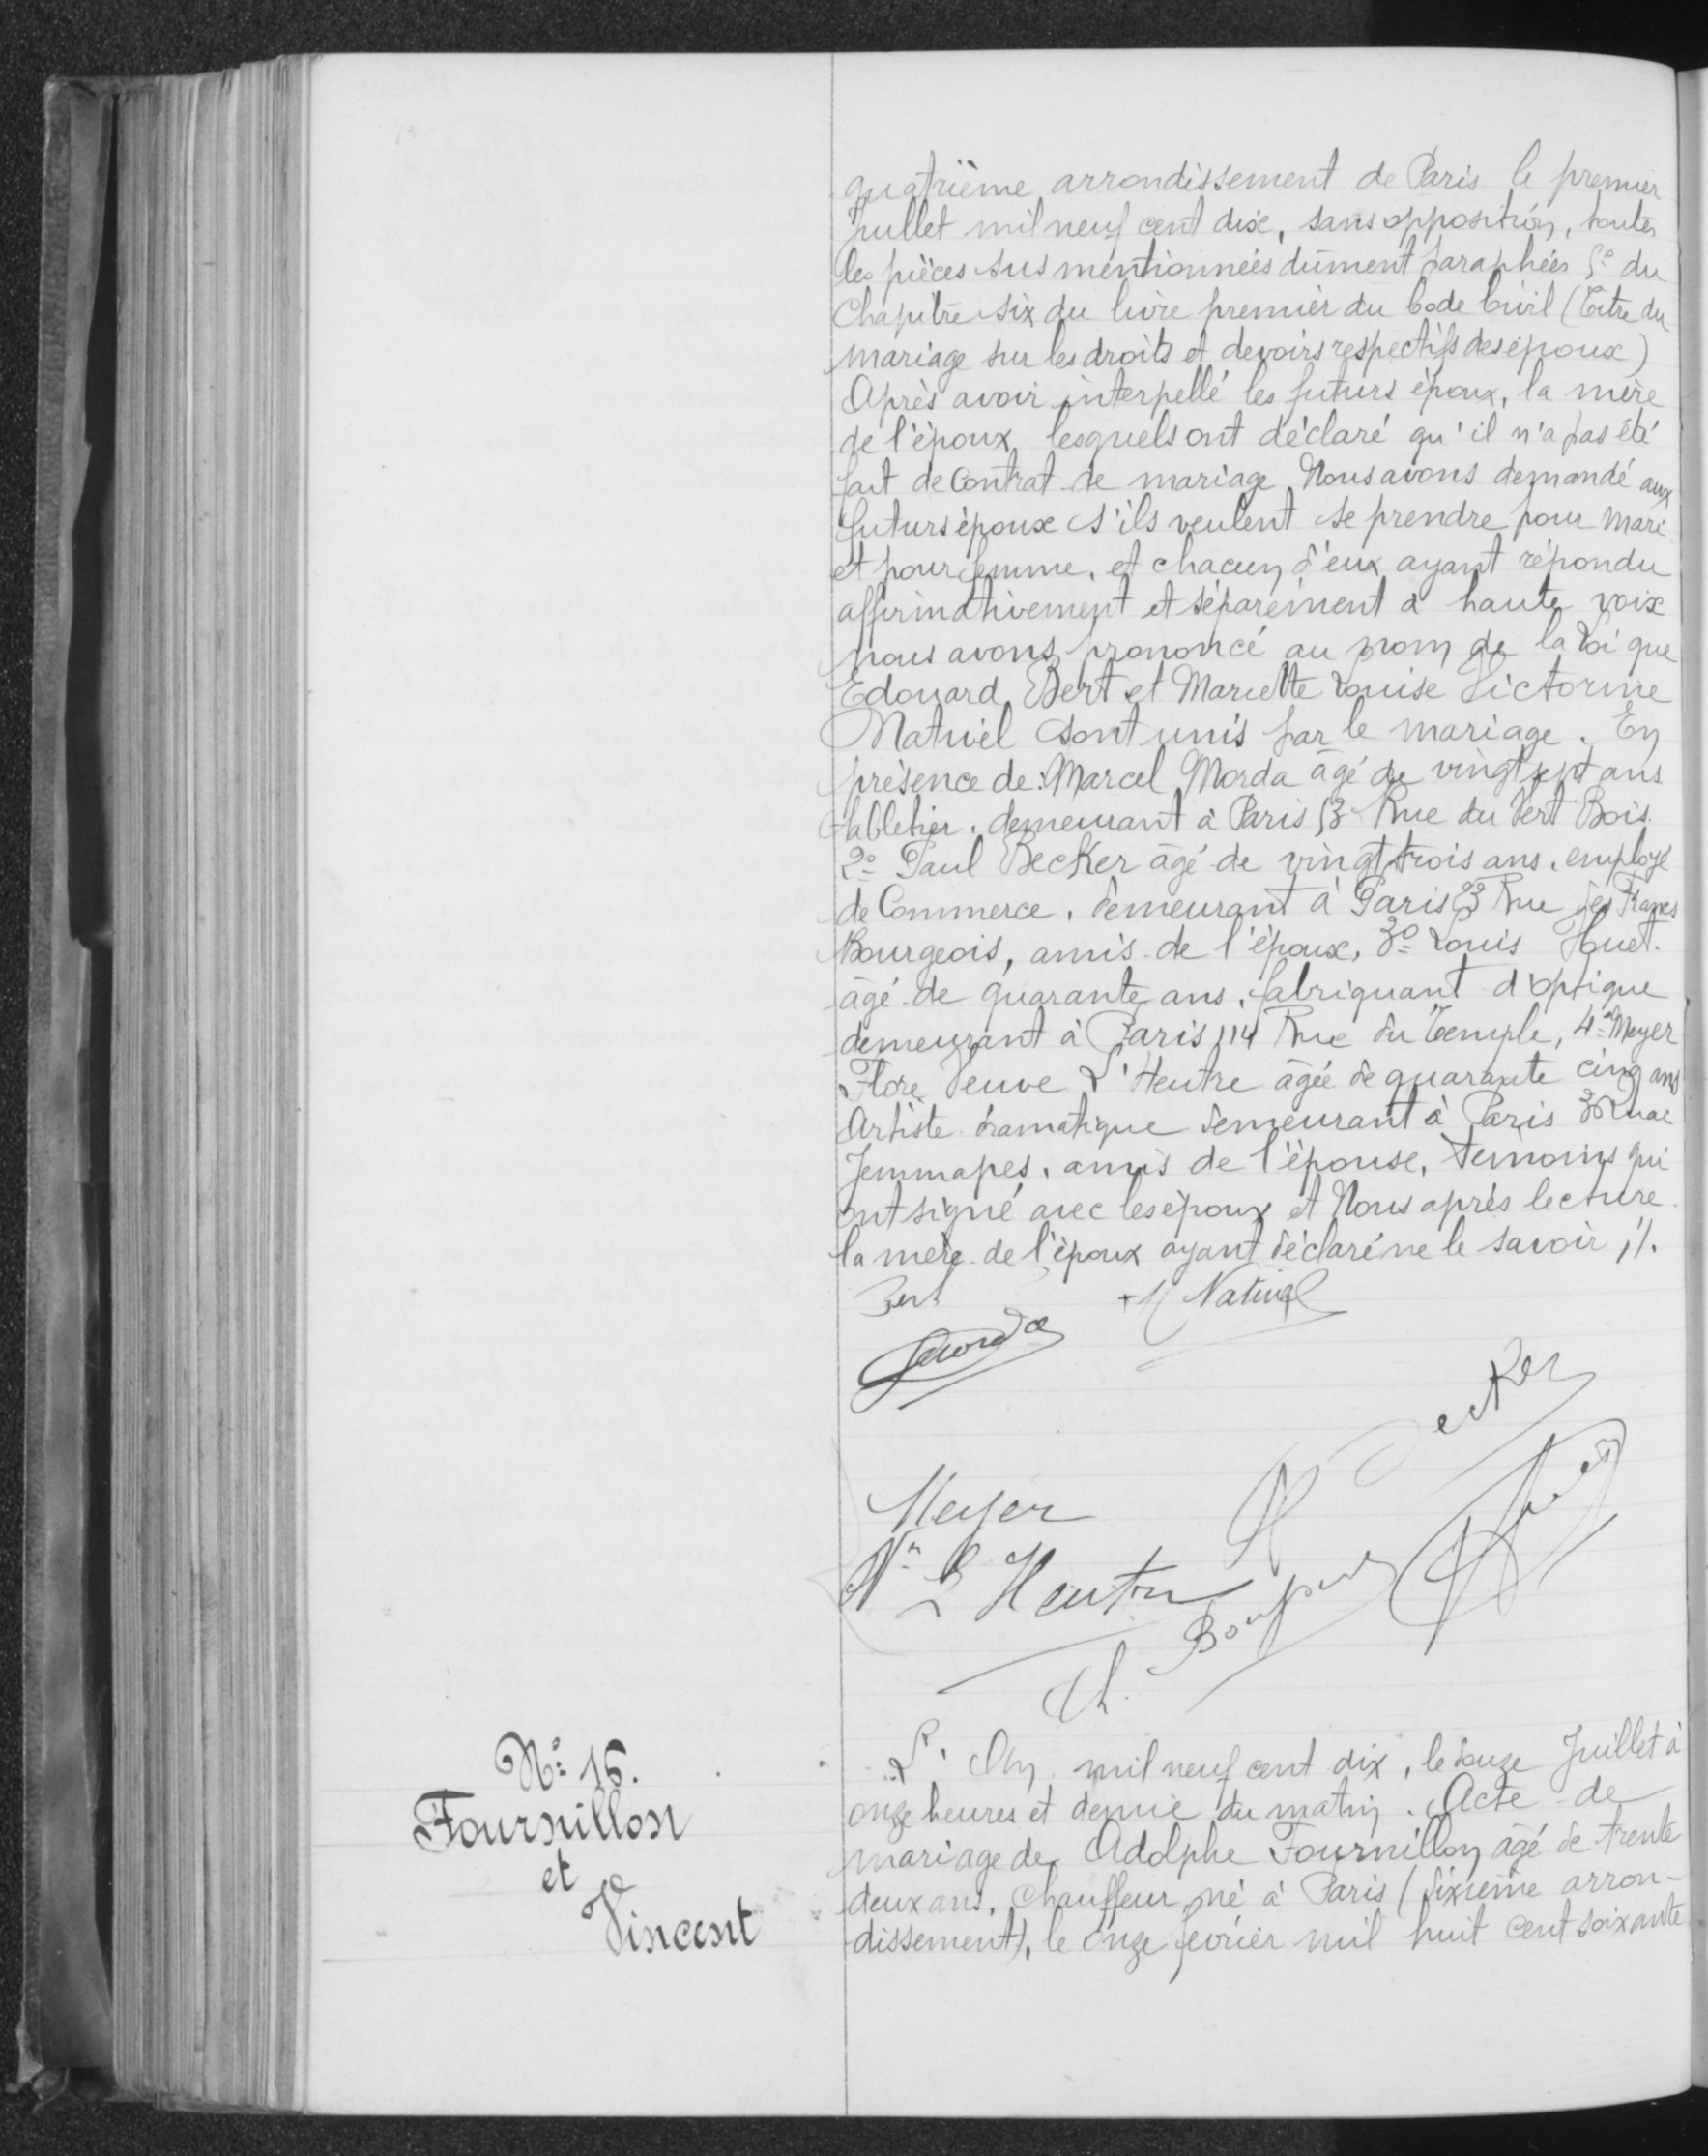quatrième arrondissement de Paris le premier
juillet mil neuf cent dix, sans opposition, toutes
les pièces sus mentionnées dûment paraphées 5° du
Chapitre six du livre premier du Code Civil (Titre du
mariage sur les droits et devoirs respectifs des époux)
Après avoir interpellé les futurs époux, la mère
de l'époux lesquels ont déclaré qu'il n'a pas été
fait de contrat de mariage Nous avons demandé aux
futurs époux s'ils veulent se prendre pour mari
et pour femme, et chacun d'eux ayant répondu
affirmativement et séparément à haute voix
nos avons prononcé au nom de la loi que
Edouard Bert et Marcette Louise Victorine
Matriel sont unis par le mariage. En
présence de Marcel Morda âgé de vingt sept ans
Glabletier, demeurant à Paris 53 Rue du Vert Bois
20-Paul Becker âgé de vingt trois ans, employé
de Commerce, demeurant à Paris 23 Rue des Francs
Bourgeois, amis de l'époux, 3°: Louis Huet
âgé de quarante ans, fabriquant d'optique
demeurant à Paris 114 Rue du Temple, 40: Meyer
Flore Veuve L'Heutre âgée de quarante cinq ans
artiste dramatique demeurant à Paris 36 rue
Jemmapes, amis de l'épouse, témoins qui
ont signé avec les époux et Nous après lecture
la mère de l'époux ayant déclaré ne le savoir ./.
N°;16
Fournillon
et
Vincent
L'an mil neuf cent dix, le douze Juillet à
onze heures et demie du matin. Acte de
mariage de Adolphe Fournillon âgé de trente
deux ans. chauffeur né à Paris sixième arron-
dissement, le onze février mil huit cent soixante
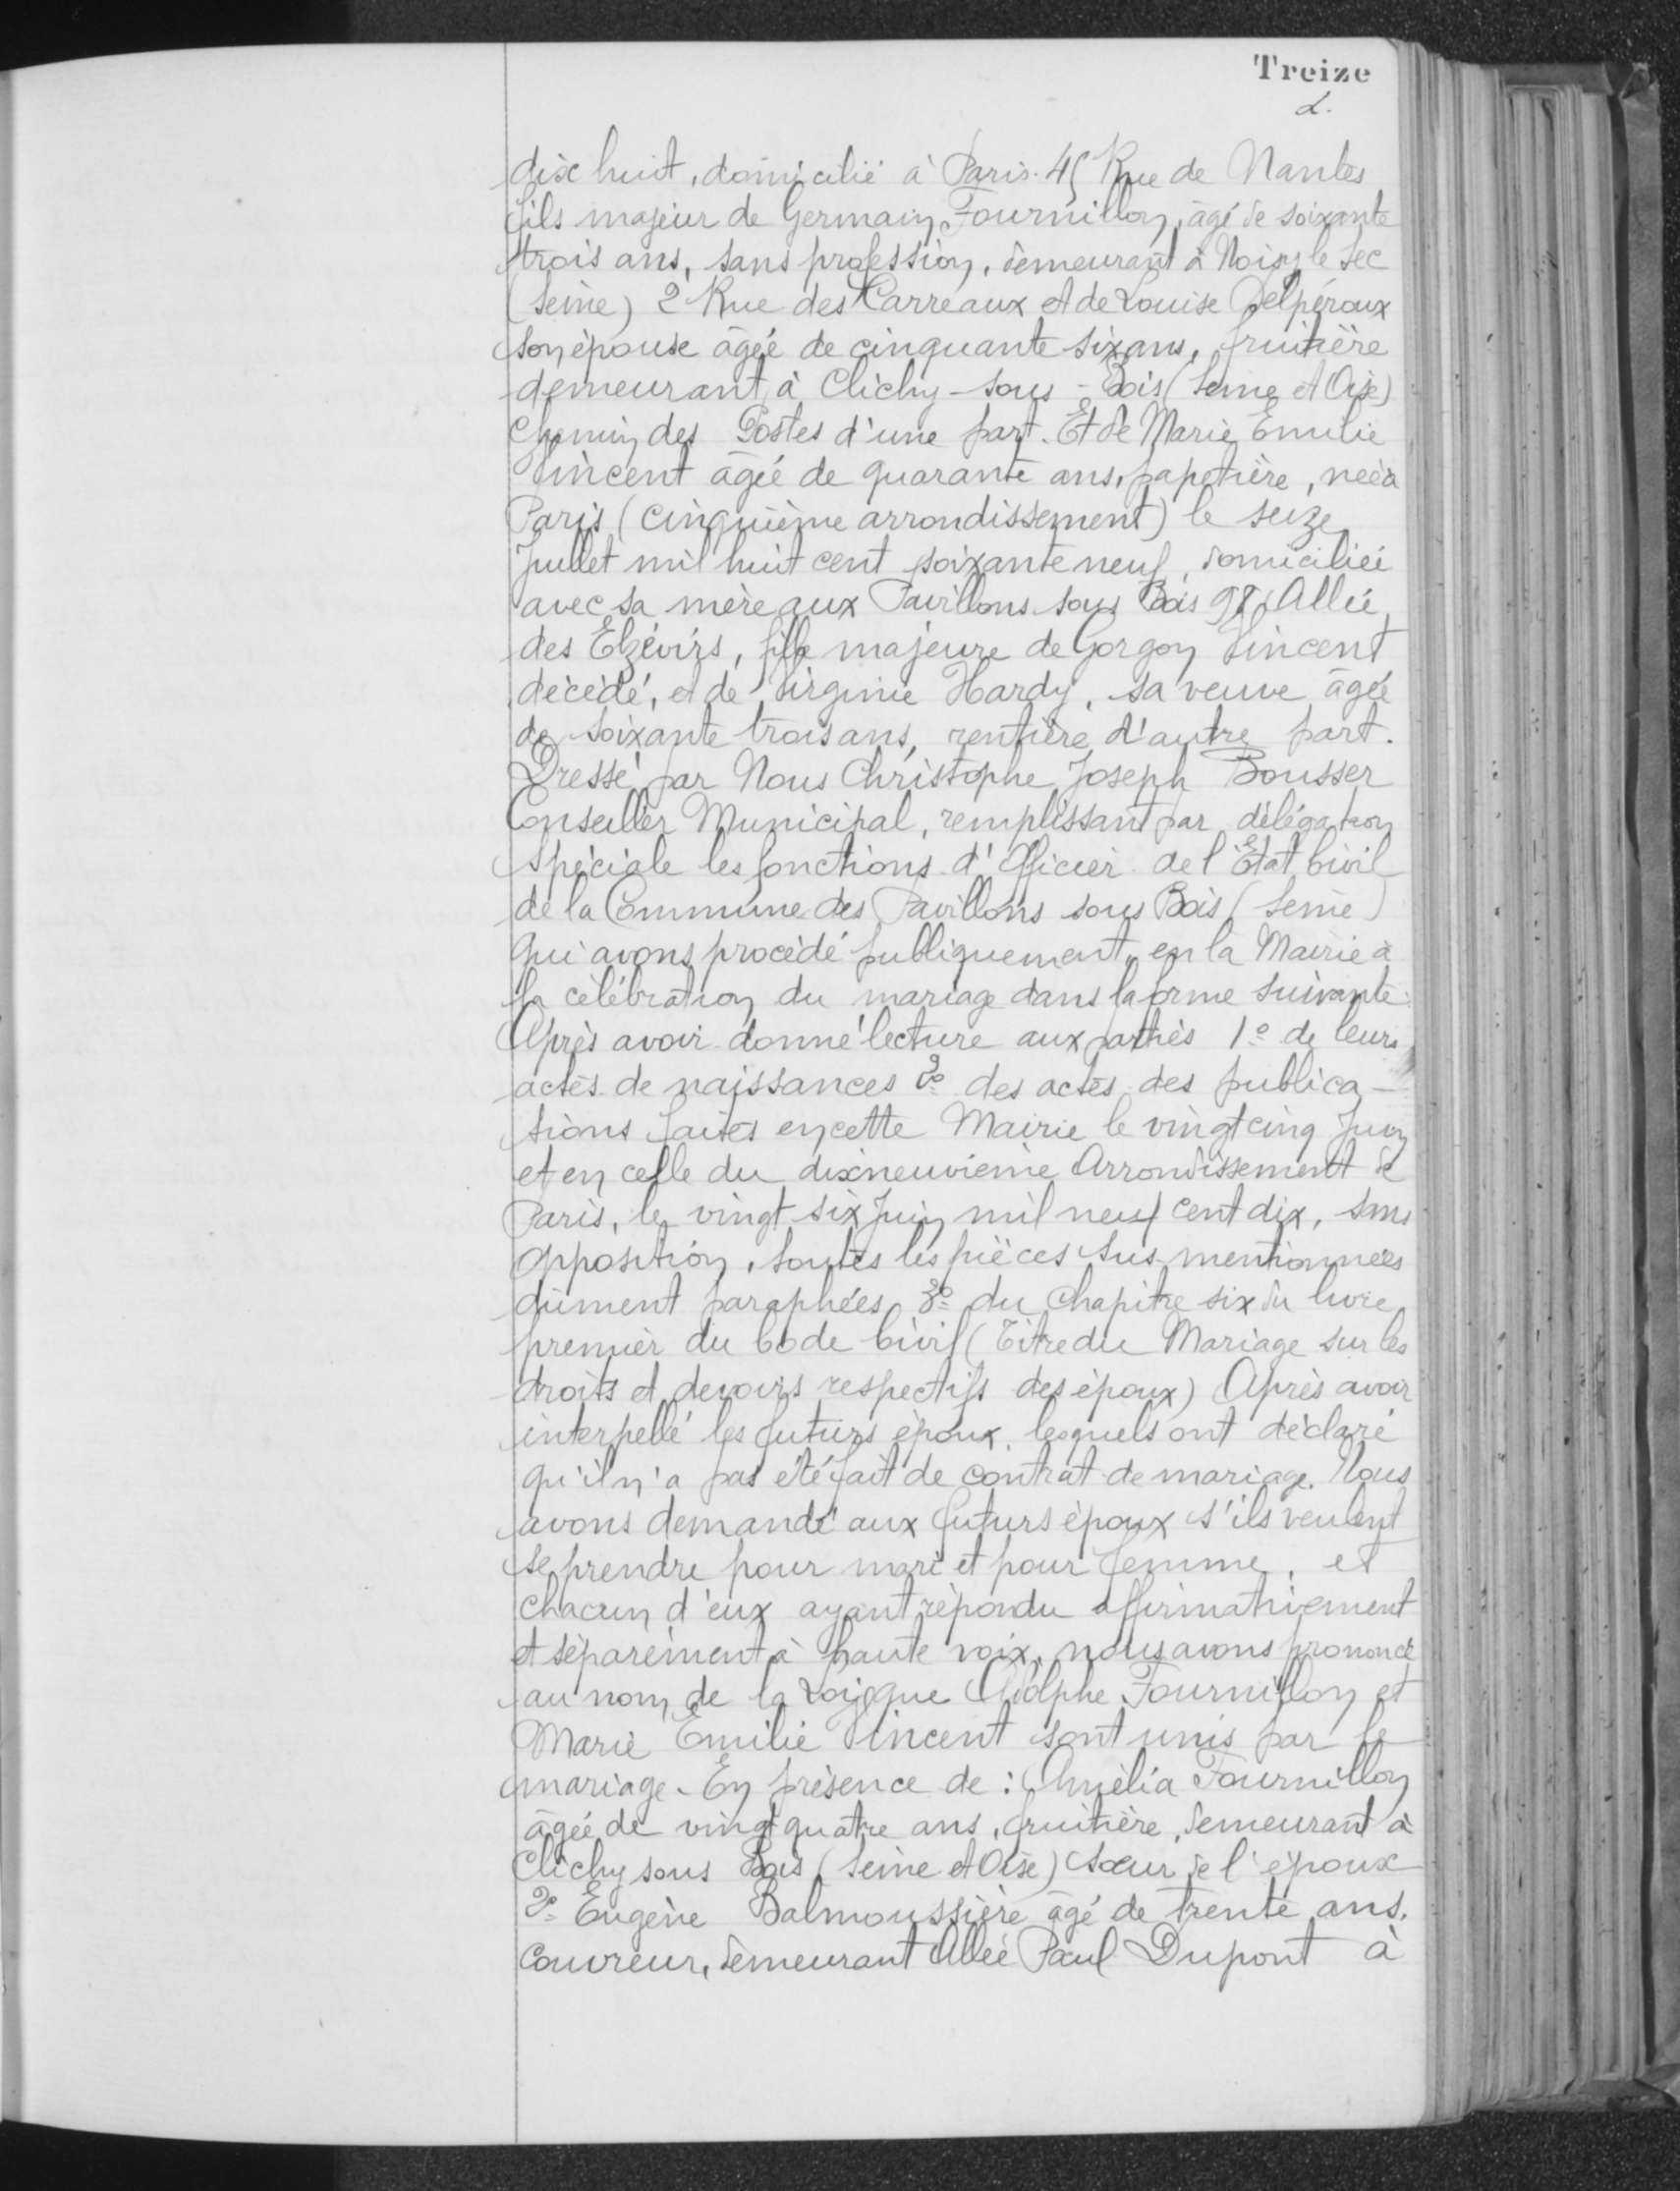dix huit, domicilié à Paris 45 Rue de Nantes
fils majeur de Germain Fournillon, âgé de soixante
trois ans, sans profession, demeurant à Noisy-le-sec
(Seine) 2 Rue des Carreaux et de Louise Delpéroux
son épouse âgée de cinquante six ans, fruitière
demeurant à Clichy sous-bois (Seine et Oise)
chemin des Postes d'une part. Et de Marie Emilie
Vincent âgée de quarante ans papetière, née à
Paris (cinquième arrondissement) le seize
Juillet mil huit cent soixante neuf, domiciliés
avec sa mère aux Pavillons sous Bois 98 Allée
des Elzévirs, fille majeur de Gorgon Vincent
décédé, et de Virginie Hardy, sa veuve âgée
de soixante trois ans, rentière d'autre part.
Dressé par Nous Christophe Joseph Bousser
Conseiller Municipal, remplissant par délégation
spéciale les fonctions d'Officier de l'Etat Civil
de la Commune des Pavillons Sous Bois (Seine)
qui avons procédé publiquement en la Mairie à
la célébration du mariage dans la forme suivante
Après avoir donné lecture aux parties 1°: de leurs
actes de naissances 2°: des actes des publica-
tions faites en cette Mairie le vingt cinq Juin
et en celle du dix neuvième arrondissement de
Paris, le vingt six Juin, mil neuf cent dix sans
opposition, toutes les pièces sus mentionnées
dûment paraphées 3°: du chapitre six du livre
premier du Code Civil (Titre du Mariage sur les
droits et devoirs respectifs des époux) Après avoir
interpellé les futurs époux lesquels ont déclaré
qu'il n'a pas été fait de contrat de mariage. Nous
avons demandé aux futurs époux s'ils veulent
se prendre pour mari et pour femme et
chacun d'eux ayant répondu affirmativement
et séparément à haute voix, nous y avons prononcé
au nom de la Loi que Adolphe Fournillon et
Marie Emilie Vincent sont unis par le
mariage. En présence de: Amélia Fournillon
âgée de vingt quatre ans, fruitière, demeurant à
Clichy sous Bois (Seine et Oise) soeur de l'époux
2°: Eugène Balmoussière âgé de trente ans,
couvreur, demeurant allée Paul Dupont à